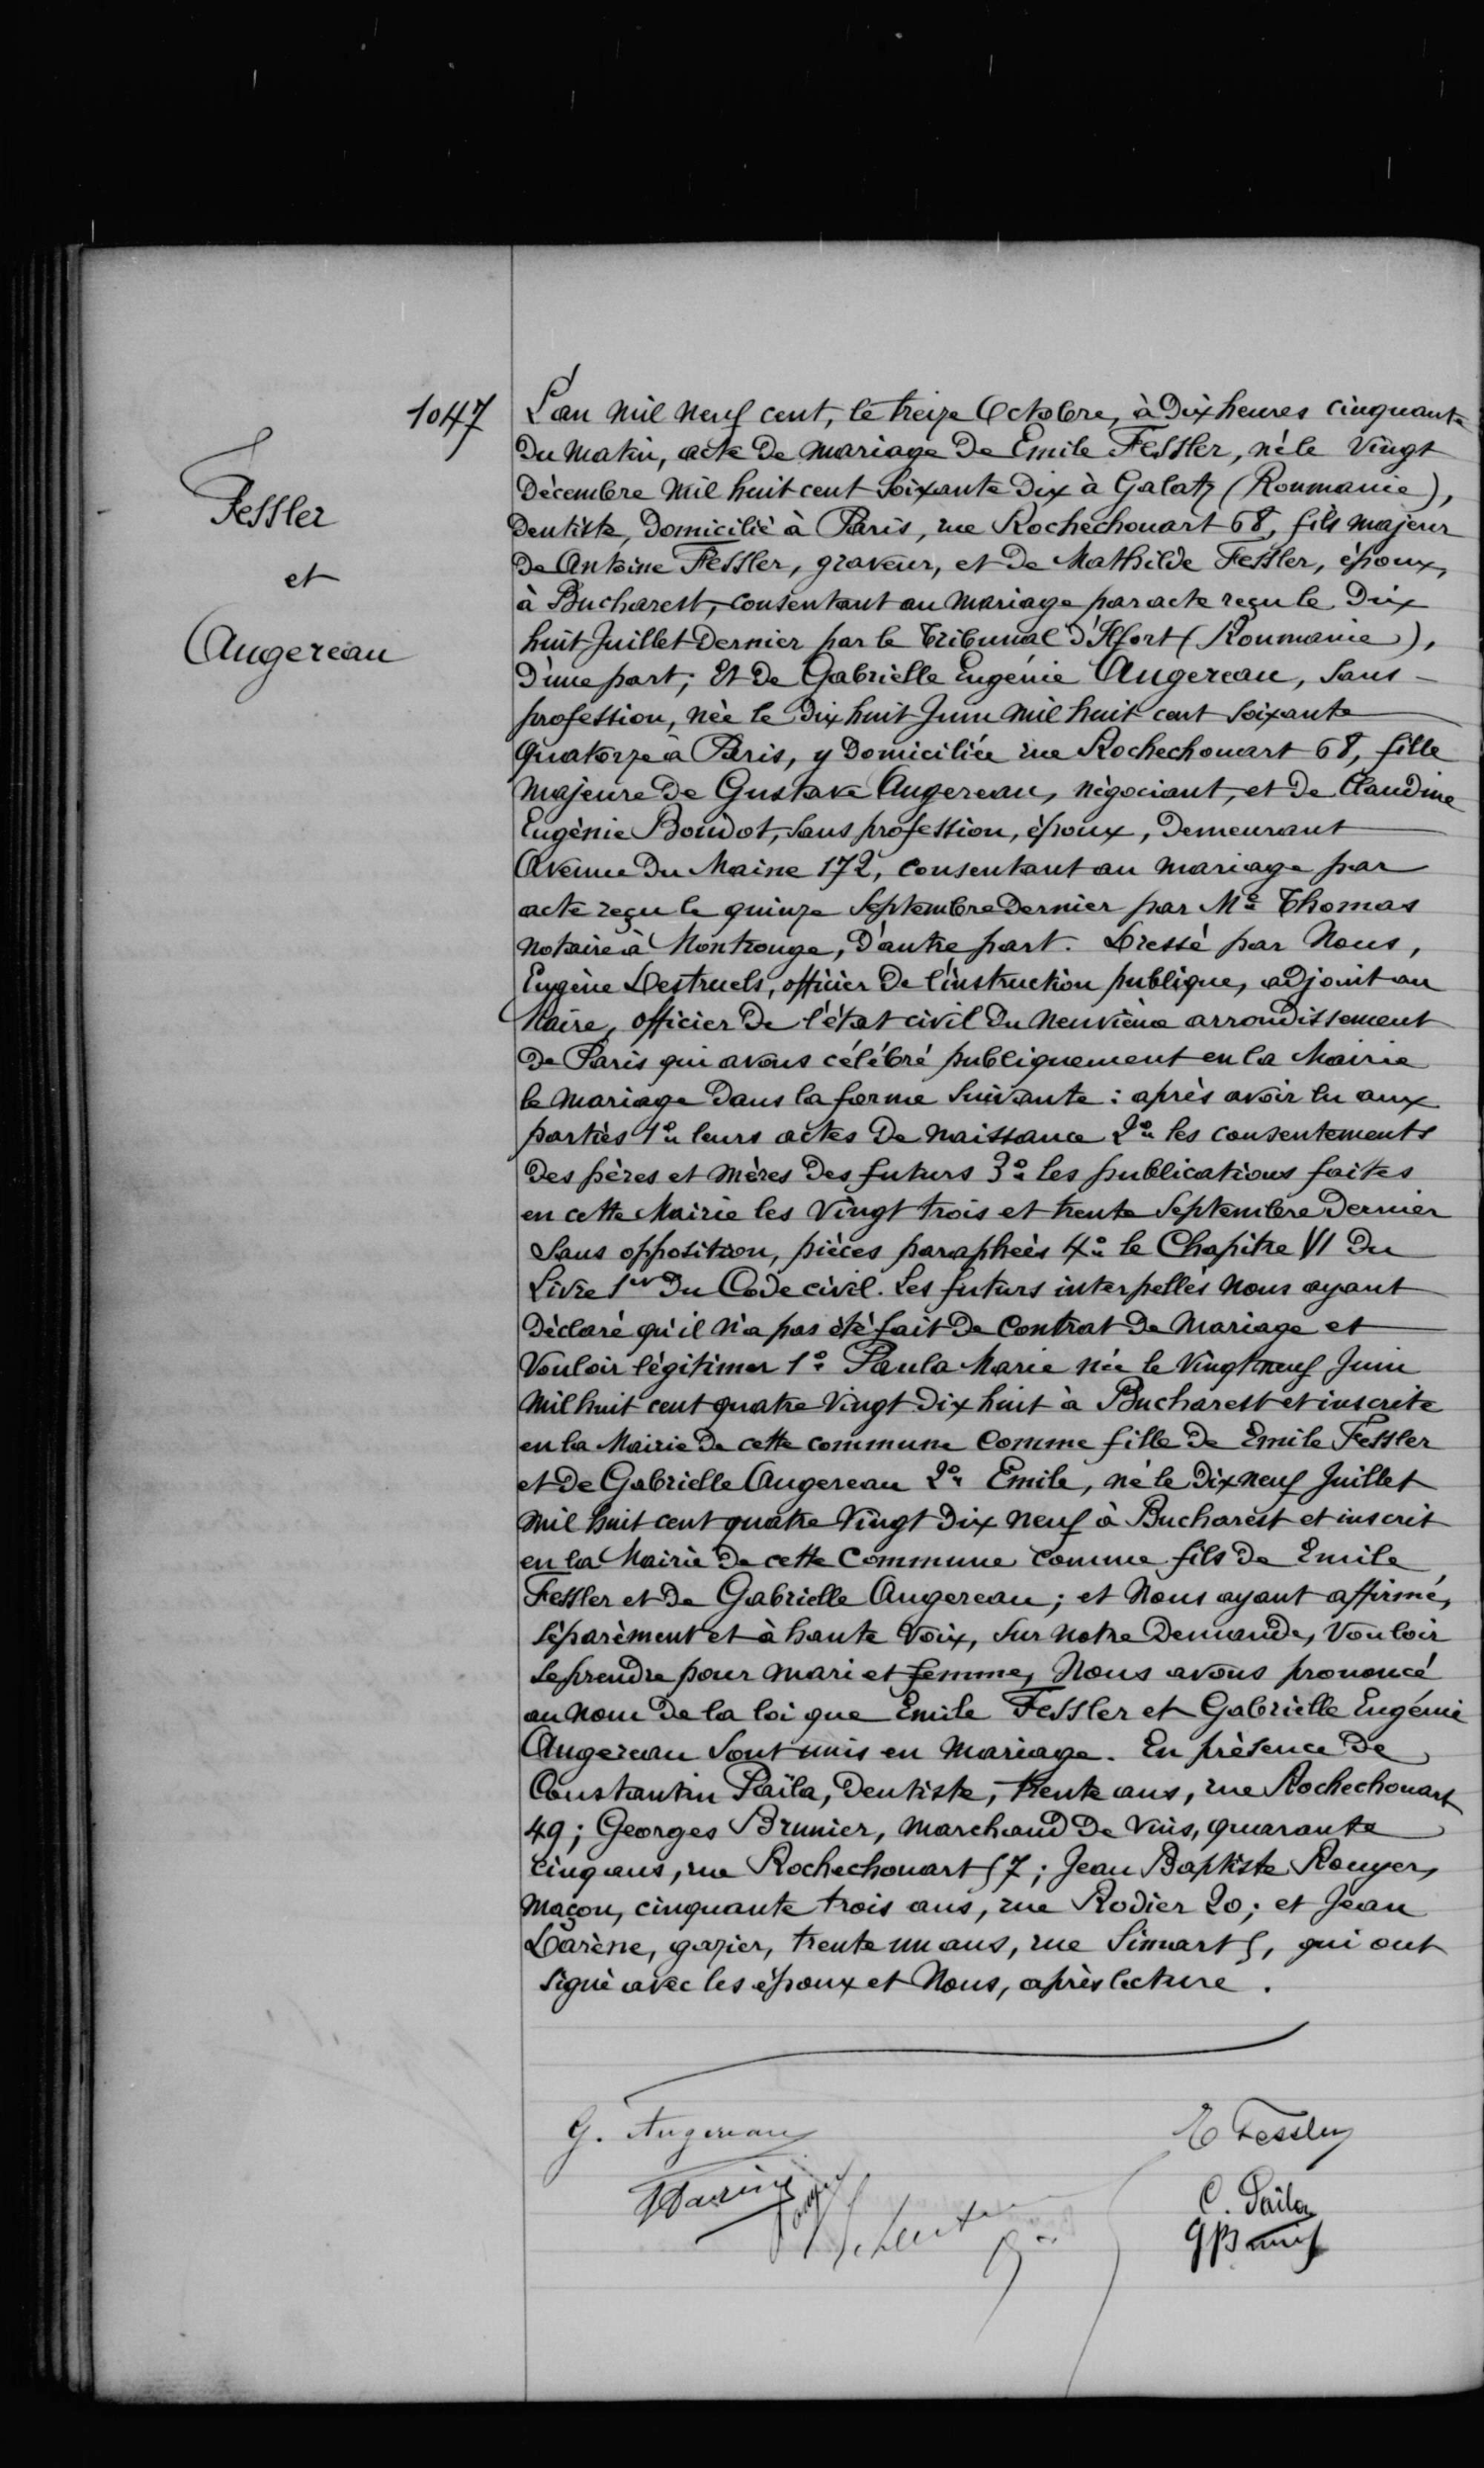Ressler
et
Augereau
L'an mil neuf cent, le treize Octobre à Dix heures, cinquante
du matin, acte de mariage de Emile Fessler, né le vingt
Dècembre mil huit cent soixante dix à Galatti (Roumanie)
Dentiste, domicilié à Paris, rue Rochechouart 68, fils majeur
de ANtoine Fessler, graveur, et de Mathile Fessler, époux,
à Bucharest, consentant au mariage par acte reçu le Dix
huit Juillet Dernier par le Tribunal d'Ilfort (Roumanie),
D'une Part; et de Gabrielle Eugénie Augereau, sans-
profession, nèe le Dix huit Juin mil huit cent soixante -
quatorze à Paris, y Domiciliée rue Rochechouart 68, fille
majeure de Gustave Augereau, négociant, et de CLaudine
Eugénie Boudot, sans profession, époux, demeurant
Avenue du Main 172, consentant au mariage par
acte reçu le quinze Septembre dernier par M° Thomas
notaire à Montrouge, D'autre part. Dressé par Nous,
Eugène Destruels, officier de l'instruction publique, adjoint au
Maire, officier de l'état civil du neuvième arrondissement
de Paris qui avons célébré publiquement en la Mairie
le Mariage dans la forme suivante: après avoir lu aux
parties 1° leurs actes de Naissance 2° les consentements
Des pères et Mères des futurs 3° les publications faites
en cette Mairie les Vingt trois et trente Septembre dernier
sans opposition, pièces paraphées 4° le Chapitre VI du
Livre 1er du Code civil. Les futurs interpellés nous ayant
déclaré qu'il n'a pas été fait de contrat de Mariage et
Vouloir légitimer 1° Paula Marie née le vingt neuf Juin
mil huit cent quatre vingt dix huit à Bucharest et inscrite
en la Mairie de cette commune comme fille de Emile Fessler
et de Gabrielle Augereau 2° Emile, né le dix neuf Juillet
mil huit cent quatre vingt dix neuf à Bucharest et inscrit
en la Mairie de cette Commune comme fils de Emile,
Fessler et de Gabrielle Augereau; et Nous ayant affirmé,
sépaèment et à haute voix, sur notre demande, vouloir
se prendre pour mari et femme, Nous avons prononcé
au nom de la loi que Emile Fessler et Gabrielle Eugénie
Augereau sont unis en Mariage. En prèsence de
Coustantin Païla, Dentiste, trente ans, rue Rochechouart
49; Georges Brunier, marchand de vins, quarante
cinq ans, rue Rochechouart 57; Jean Baptiste Rouyer,
Maçou, cinquante trois ans, rue Rodier 20; et Jean
Larène, gazier, trente un ans, rue Simart 51, qui ont
signé avec les époux et Nous, après lecture.
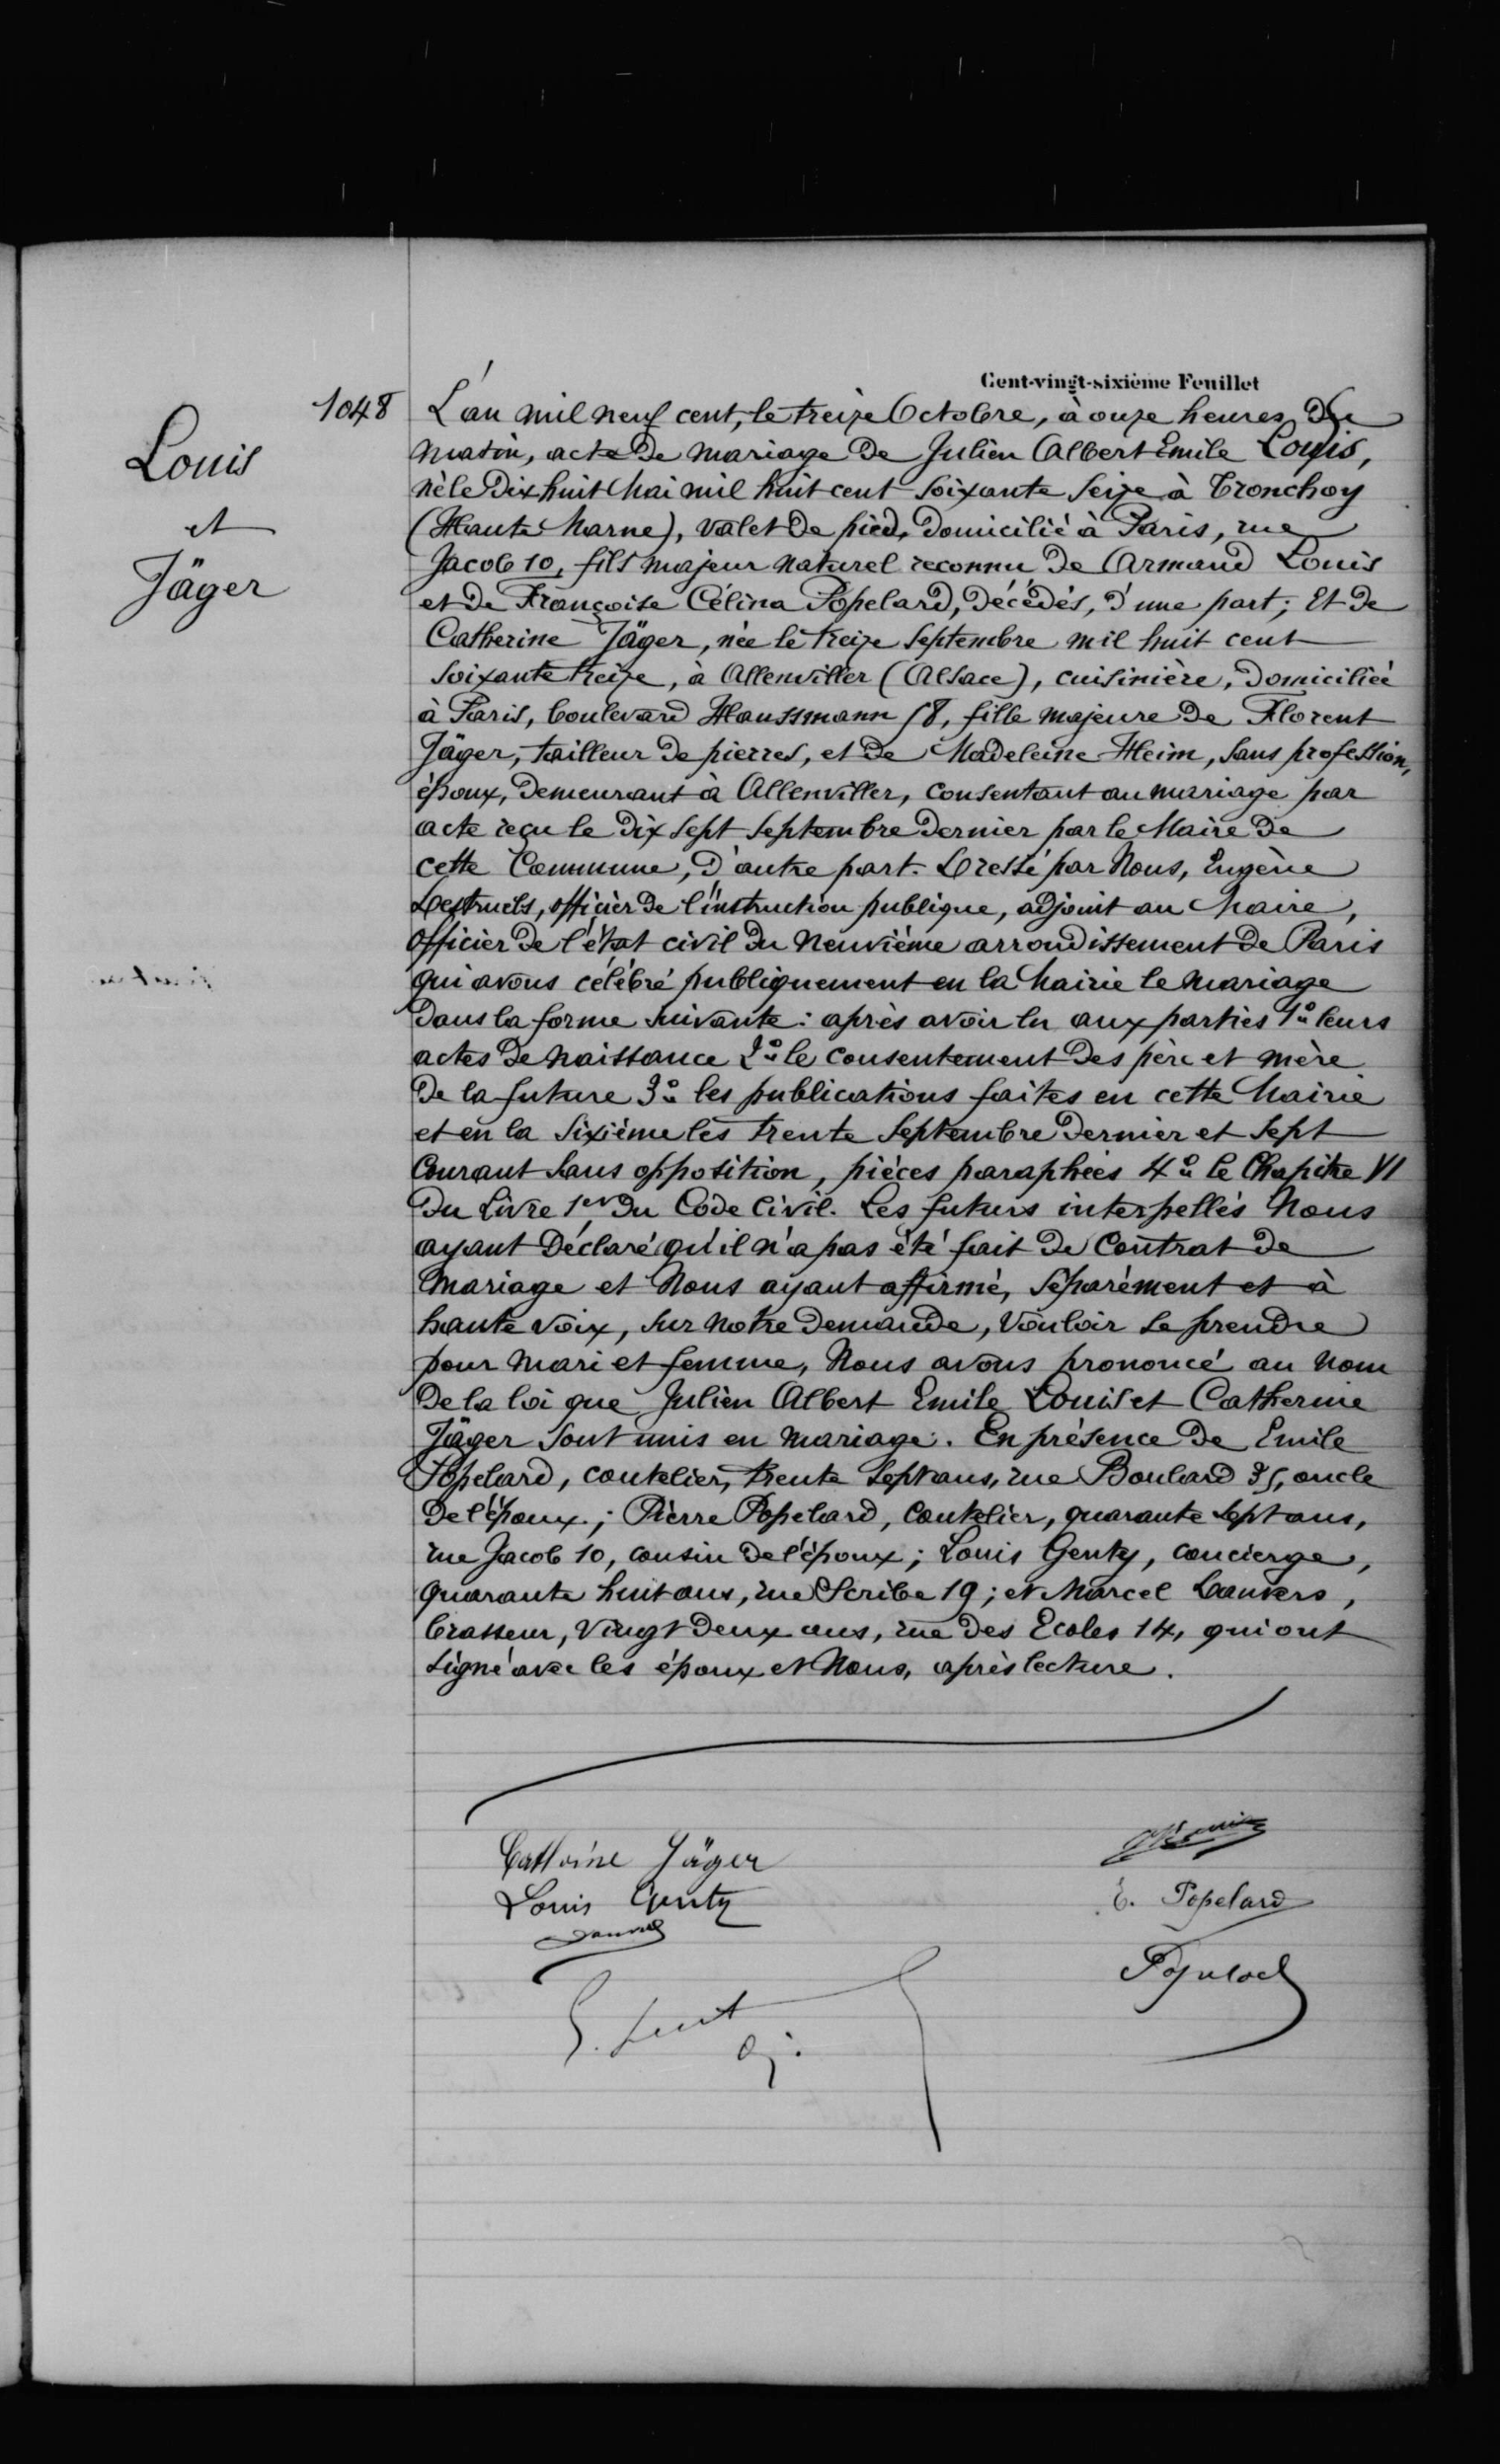1048
Louis
et
Jäger
L'an mil neuf cent, le treize Octobre, à onze heures du
Matin, acte de mariage de Julien Albert Emile Louis,
né le Dix huit mai mil huit cent soixante seize à Tronchoy
(Haute Marne), valet de pied, domicilié à Paris, rue
Jacob 10, fils majeur naturel reconnu de Armand Louis
et de François Célina Popelard, décédés, d'une part; Et de
Catherine Jäger, née le treize Septembre mil huit cent
soixante treize, à alleniviller (Alsace), cuisinière, domiciliée
à Paris, boulevard Haussmann 58, fille majeure de Florent
Jäger, tailleur de pierres, et de Madeleine Heim, sans profession
époux, demeurant à Allenviller, consentant au mariage par
acte reçu le dix sept septembre dernier par le Maire de
Cette commune, d'autre part. Dressé par Nous, Eugène
Lestruels, officier de l'instruction publique, adjoint au Maire,
officier de l'état civil du Neuvième arrondissement de Paris
qui avons célébré publiquement en la Maire le Mariage
Dans la forme suivante: après avoir lu aux parties 4° leurs
actes de naissance 2° le consentement des père et mère
de la future 3° les publications faites en cette Mairie
et en la sixième les trente Septembre dernier et sept
courant leur opposition, pièces paraphées 4° le Chapitre VI
du Livre 1er du Code Civil. Les futurs interpellés Nous
ayant déclaré qu'il n'a pas été fait de Contrat de
Mariage et Nous ayant affirmé, séparèment et à
haute voix, sur notre demande, vouloir se prendre
pour mari et femme, Nous avons prononcé au nom
de la loi que Julier Albert Emile Louis et Catherine
Jäger sont unis au mariage: En présence de Emile
Hopelard, coutelier, trente sept ans, rue Boulard 35 oncle
De l'époux; Pierre Popelard, coutelier, quarante sept ans,
rue Jacob 10, cousin de l'époux; Louis Genty, concierge,
quarante huit ans, rue Scribe 19; et Marcel Lausers,
brasseur, Vingt deux ans, rue des Ecoles 14, qui ont
signé avec les époux et Nous, après lecture.